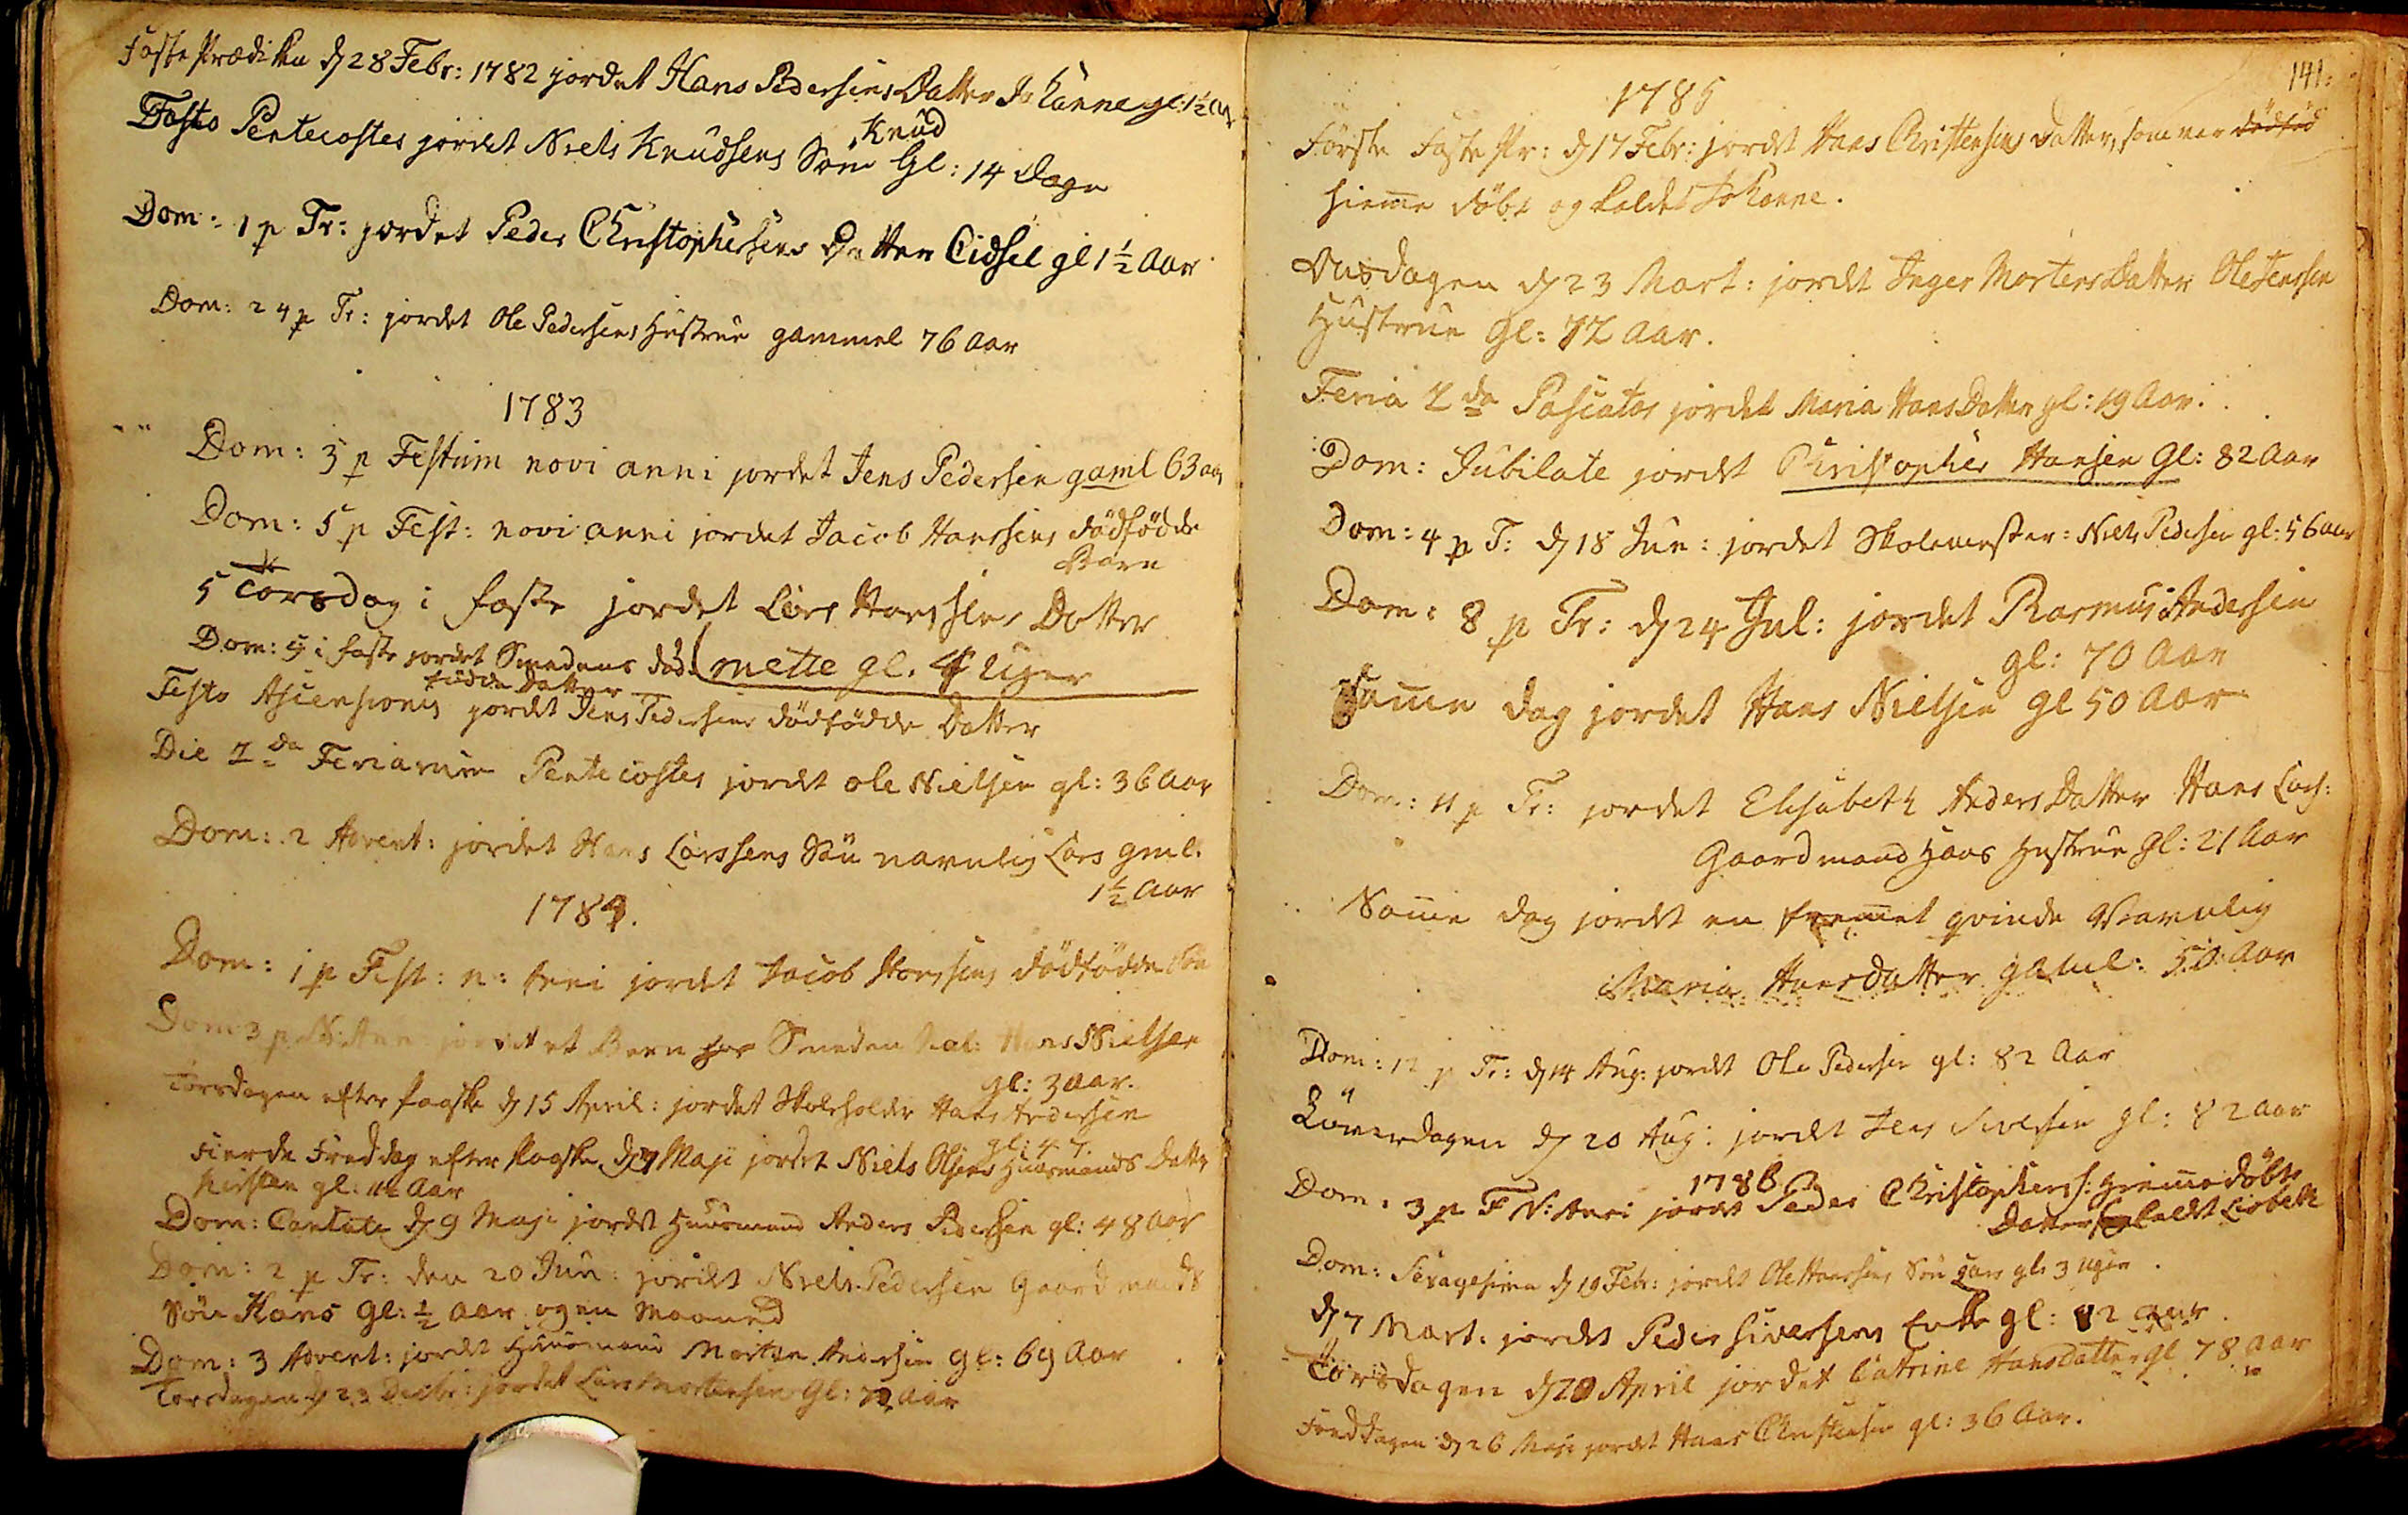Faste Prædike d 28 Febr: 1782 jordet Hans Pedersens Datter Johanne gl:1½ Aar
Knud
Festes Pentecostes jordet Niels Knudsens Søn Gl: 14 dage
Dom: 1 p Tr: jordet Peder Christophersens Datter Cidsel gl 1½ Aar
Dom: 24 p Tr: jordet Ole Pedersens Hustrue gammel 76 Aar
1783
Dom: 3 p Festum novi Anni jordet Jens Pedersen gamml 63 Aar
Dom: 5 p Fest: novi Anni jordet Jacob Hanssens dødfødde
Barn
5 Torsdag i Faste jordet Lars Hanssens Datter
Mette gl: 4 Uger
Dom: 5 i Faste jordet Smedens død
fødde Datter
Festo Ascensionis jordet Jens Pedersens dødfødde Datter
Die 2da Feriarum Pentecostes jordet Ole Nielsen gl: 36 Aar
Dom: 2 Advent: jordet Hans Larssens Søn navnlig Lars gml:
1½ Aar
1784.
Dom: 1 p Fest: n: Anni jordet Jacob Hanssens dødfødde Søn
Dom: 3 p N: Anni: jordet et Barn hos Smeden Sal: Hans Nielsen
gl: 3 Aar
Torsdagen efter Paaske d 15 April: jordet Skoleholder Hans Andersen
gl: 47.
Fierde Freddag efter Paaske d 7 Maji jordet Niels Olsens Huusmands Datter
Kirsten gl: 11½ Aar
Dom: Cantate d 9 Maji jordet Huusmand Anders Pedersen gl: 48 Aar
Dom: 2 p Tr: den 20 Jun: jordet Niels Pedersen Gaardmands
Søn Hans gl: ½ Aar og en Maaned
Dom: 3 Advent: jordet Huusmand Morten Andersen gl: 69 Aar
Torsdagen d 23 Decbr: jordet Lars Mortensen Gl: 72## Aar
141
1785
Første Faste Pr: d 17 Febr: jordet Hans Christensens Datter, som var dødfød
hieme døbt og kaldet Johanne.
Onsdagen d 23 Mart: jordet Inger Mortens Datter Ole Jenssen
Hustrue Gl: 72 Aar
Feria 2da Pascatos jordet Maria Hans Datter gl: 19 Aar.
Dom: Jubilate jordet Christopher Hansen Gl: 82 Aar
Dom: 4 p T: d 18 Jun: jordet Skolemester = Niels Pedersen gl: 56 Aar
Dom: 8 p Tr: d 24 Jul: jordet Rasmus Andersen
gl: 70 Aar
Samme dag jordet Hans Nielsen gl 50 Aar
Dom: 11 p Tr: jordet Elisabeth Anders Datter Hans Lars:
Gaardmand Hans Hustrue gl: 21 Aar
Same dag jordet en fremmet qvinde Navnlig
Maria Hansdatter gaml: 50 Aar
Dom: 12 p Tr: d 14 Aug: jordet Ole Pedersen gl: 82 Aar
Løverdagen d 20 Aug: jordet Jens Siversen gl: 82 Aar
1786
Dom: 3 p F N: Anni jordet Peder Christopherss: Hiemedøbte
Datter kaldet Lisbeth
Dom: Sexagesima d 19 Febr: jordet Ole Hanssens Søn Lars gl: 3 Uger
d 7 Mart: jordet Peder Siversens Enke gl: 82 Aar
Torsdagen d 20## April jordet Catrine Hansdatter gl 78 Aar
Freddagen d 26 Maji jordet Hans Christensen gl: 36 Aar.
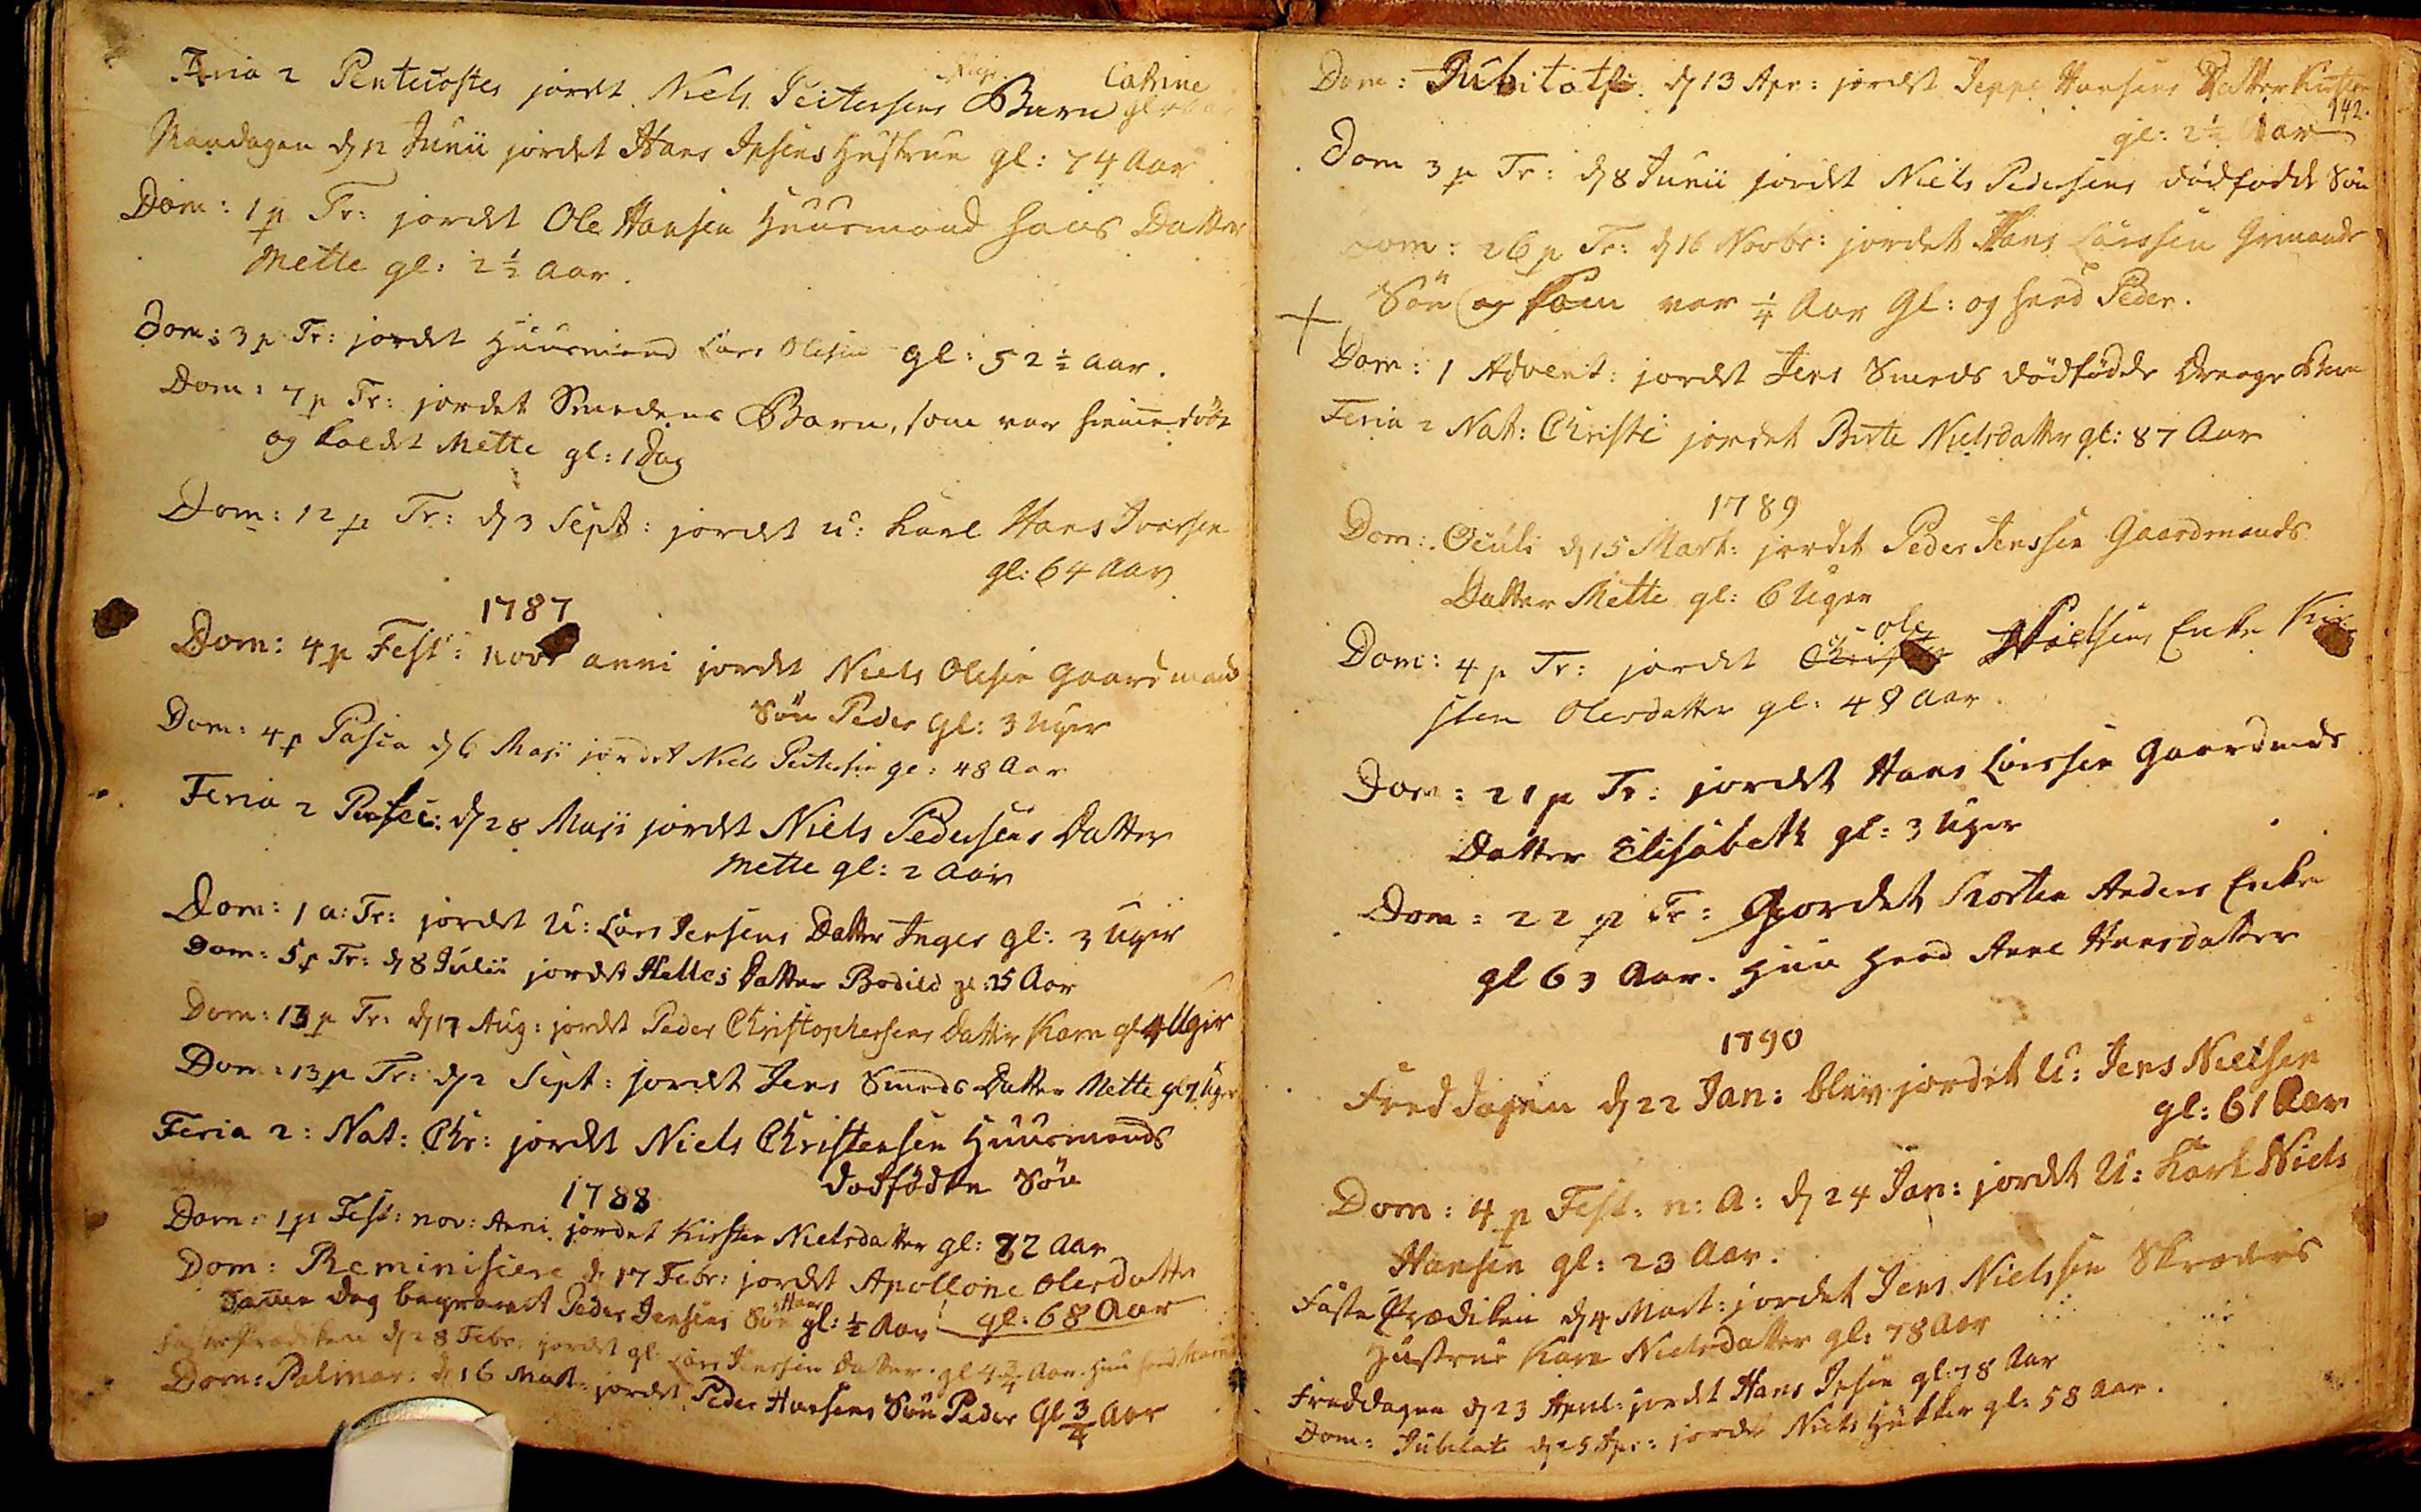Pige
Catrine
Feria 2 Pentecostes jordet Niels Peitersens Barn gl 4 Aar
Mandagen d 12 Junii jordet Hans Ipsens Hustrue gl: 74 Aar
Dom: 1 p Tr: jordet Ole Hansen Huusmand hans Datter
Mette gl: 2½ Aar.
Dom: 3 p Tr: jordet Huusmand Lars Olesen gl: 52½ Aar
Dom: 7 p Tr: jordet Smedens Barn, som var hiemedøbt
og kaldet Mette gl:1 Dag
Dom: 12 p Tr: d 3 Sept: jordet u: karl Hans Ivarsen
gl: 64 Aar
1787
Dom: 4 p Fest: novi anni jordet Niels Olesen Gaardmands
Søn Peder gl: 3 Uger
Dom: 4 p Pasca d 6 Maji jordet Niels Peitersen gl: 48 Aar
Feria 2 Pentec: d 28 Maji jordet Niels Pedersens Datter
Mette gl: 2 Aar
Dom: 1 a: Tr: jordet U: Lars Jensens Datter Inger gl: 3 Uger
Dom: 5 p Tr: d 8 Julii jordet Helles Datter Bodild gl: 25 Aar
Dom: 13 p Tr: d 17 Aug: jordet Peder Christophersens Datter Karn gl 4 Uger
Dom: 13 p Tr: d 2 Sept: jordet Jens Smeds Datter Mette gl 2 Uger
Feria 2: Nat: Chr: jordet Niels Christensen Huusmands
dødfødde Søn
1788
Dom: 1 p Fest: nov: Anni jordet Kirsten Nielsdatter gl: 82 Aar
Dom: Reminiscere d 17 Febr: jordet Apollone Olesdatter
gl: 68 Aar
Hans
Same Dag begravet Peder Jensens Søn gl: ½ Aar
Faste Prædiken d 28 Febr: jordet gl: Lars Jenssens Datter gl 4 ¾ Aar. Hun heed Marn
Dom: Palmar: d 16 Mart jordet Peder Hansens Søn Peder gl ¾ Aar
142.
Dom: Jubilate. d 13 Apr: jordet Jeppe Hansens Datter Kirsten
gl: 2½ Aar
Dom: 3 p Tr: d 8 Junii jordet Niels Pedersens dødfødde Søn
Dom: 26 p Tr: d 16 Novbr: jordet Hans Larssen Grmands
Søn og han var ¼ Aar gl: og heed Peder.
Dom: 1 Advent: jordet Jens Smeds dødfødde Drenge Barn
Feria 2 Nat: Christi jordet Birte Nielsdatter gl: 87 Aar
1789
Dom: Oculi d 15 Mart: jordet Peder Jenssen Gaardmands
Datter Mette gl: 6 Uger
Ole
Dom: 4 p Tr: jordet Christen Nielsens Enke Kir
sten Olesdatter gl: 48 Aar.
Dom: 21 p Tr: jordet Hans Larssen Gaardmands
Datter Elisabeth gl: 3 Uger
Dom: 22 p Tr: jordet Morten Anders Enke
gl 63 Aar. Hun hedd Anne Hansdatter
1790
Freddagen d 22 Jan: blev jordet U: Jens Nielsen
gl: 61 Aar
Dom: 4 p Fest: n: A: d 24 Jan: jordet U:karl Niels
Hansen gl: 23 Aar.
Faste Prædiken d 4 Mart: jordet Jens Nielsen Skræders
Hustrue Karn Nielsdatter gl: 78 Aar
Freddagen d 23 April: jordet Hans Ipsen gl: 78 Aar.
Dom: Jubilate d 25 Apr: jordet Niels Hukker gl: 58 Aar.